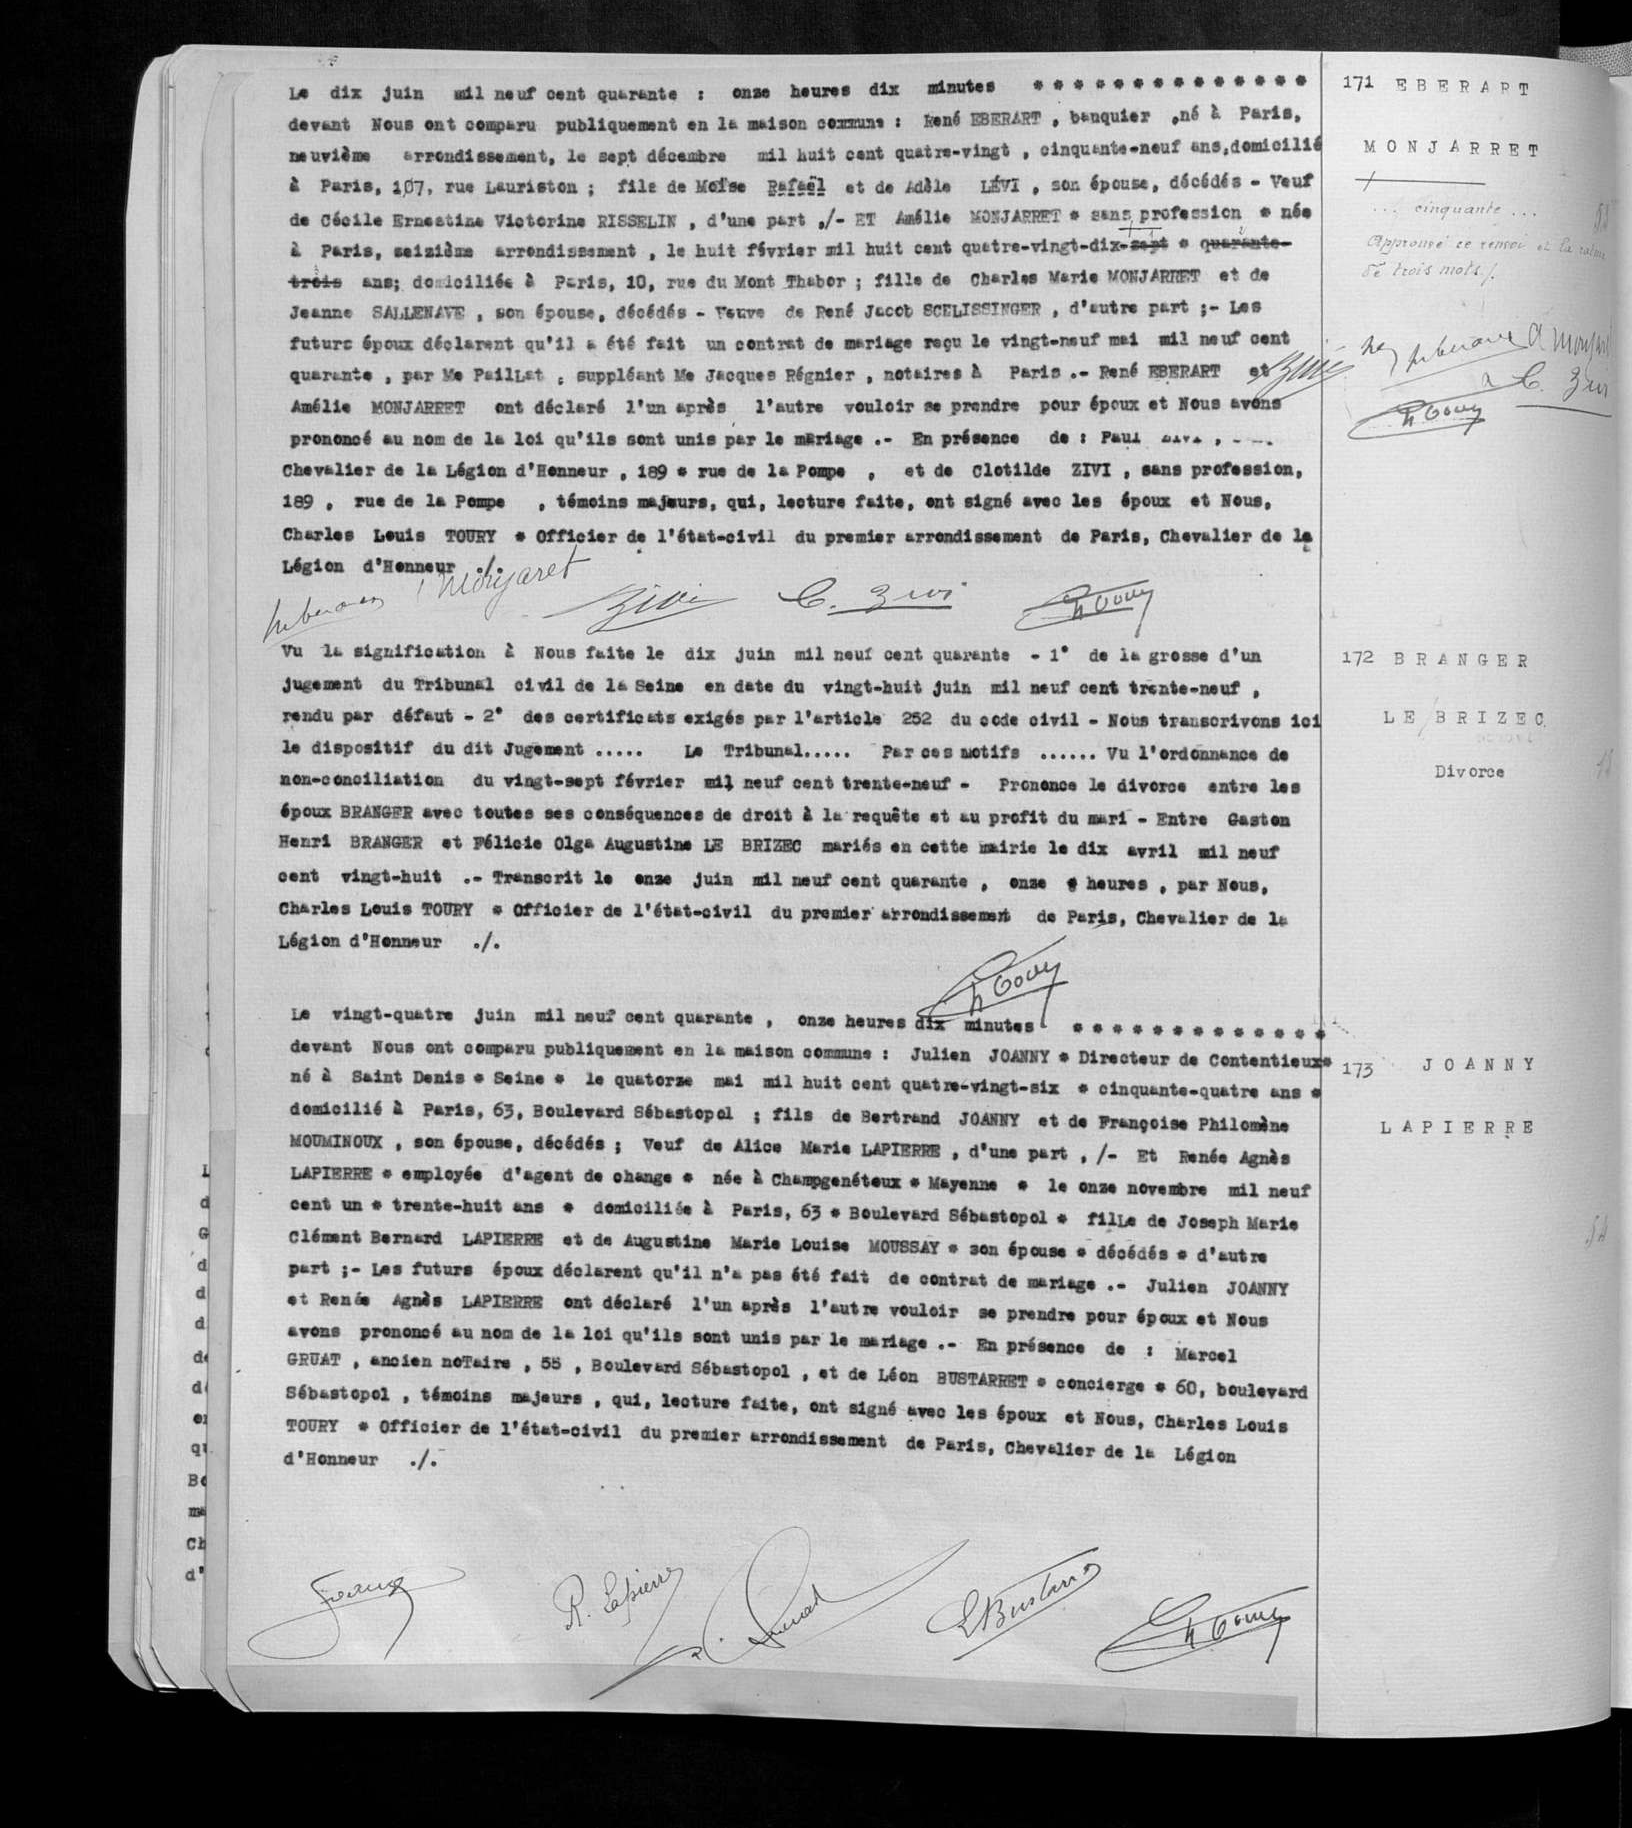le dix juin mil neuf cent quarante: onze heures dix minutes ****
devant Nous ont comparu publiquement en la maison commune: René EBERART, banquier, né à Paris,
neuvième arrondissement, le sept décembre mil huit cent quatre-vingt, cinquante-neuf ans, domicilié
à Paris, 107, rue Lauriston; fils de Moïse Rafaël et de Adèle LEVU, son épouse, décédés-Veuf
de Cécile Ernestine Victorine RISSELIN, d'une part ,/-ET Amélie MONJARRET * sans profession * née
à Paris, sixième arrondissement, le huit février mil huit cent quatre-vingt-dix-sept *
quarante-trois ans; domiciliée à Paris, 10, rue du Mont Thabor; fille de Charles Marie MONJARRET et de
Jeanne SALLENAVE, son épouse, décédés-Fouve de Réne Jacob SCELISSINGER, d'autre part ;-Les
futurs époux déclarent qu'il a été fait un contrat de mariage reçu le vingt-neuf mai mil neuf cent
quarante, par Me PailLat, suppléant Me Jacque Régnier, notaires à Paris .-René EBERART et
Amélie MONJARRET ont déclaré l'un après l'autre vouloir se prendre pour époux et Nous avons
prononcé au nom de la loi qu'ils sont unis par le mariage .-En présence de: Paul,
Chevalier de la Légion d'Honneur, 189 * rue de la Pompe, et de Clotilde ZIVI, sans profession,
189, rue de la Pompe, témoins majeurs, qui, lecture faite, ont signé avec les époux et Nous,
Charles Louis TOURY * Officier de l'état-civil du premier arrondissement de Paris, Chevalier de la
Légion d'Honneur ./.
Vu la signification à Nous faite le dix juin mil neuf cent quarante-1° de la grosse d'un
jugement du Tribunal civil de la Seine en date du vingt-huit juin mil neuf cent trente-neuf,
rendu par défaut-2° des certificats exigés par l'article 252 du code civil-Nous transcrivons ici
le dispositif du dit Jugement.... Le Tribunal..... Par ces motifs... Vu l'ordonnance de
non-conciliation du vingt-sept février mil neuf cent trente-neuf-Prononce le divorce entre les
époux BRANGER avec toutes ses conséquences de droit à la requête et au profit du mari-Entre Gaston
Henri BRANGER et Félicie Olga Augustine LE BRIZEC mariés en cette mairie le dix avril mil neuf
cent vingt-huit .-Transcrit le onze juin mil neuf cent quarante, onze heures, par Nous,
Charles Louis TOURY * Officier de l'état-civil du premier arrondissement de Paris, Chevalier de la
Légion d'Honneur ./.
le vingt-quatre juin mil neuf cent quarante, onze heures dix minutes ****
devant Nous ont comparu publiquement en la maison commune: Julien JOANNY * Directeur de Contentieux *
né à Saint Denis * Seine * le quatorze mai mil huit cent quatre-vingt-six * cinquante-quatre ans *
domicilié à Paris, 63, Boulevard Sébastopol; fils de Bertrand JOANNY et de Françoise Philomène
MOUMINOUX, son épouse, décédés; Veuf de Alice Marie LAPIERRE, d'une part ,/-ET Rénee Agnès
LAPIERRE * employée d'agent de change * née à Chamgenéteux * Mayenne * le onze novembre mil neuf
cent un * trente-huit ans * domiciliée à Paris, 63 * Boulevard Sébastopol * fille de Joseph Marie
Clément Bernard LAPIERRE et de Augustine Marie Louise MOUSSAY * son épouse * décédés * d'autre
part ;-Les futurs époux déclarent qu'il n'a pas été fait de contrat de mariage .-Julien JOANNY
et Renée Agnès LAPIERRE ont déclaré l'un après l'autre vouloir se prendre pour époux et Nous
avons prononcé au nom de la loi qu'ils sont unis par le mariage .-En présence de: Marcel
GRUAT, ancien notaire, 55, Boulevard Sébastopol, et de Léon BUSTARRET * concierge * 60, boulevard
Sébastopol, témoins majeurs, qui, lecture faite, ont signé avec les époux et Nous, Charles Louis
TOURY * Officier de l'état-civil du premier arrondissement de Paris, Chevalier de la Légion
d'Honneur ./.
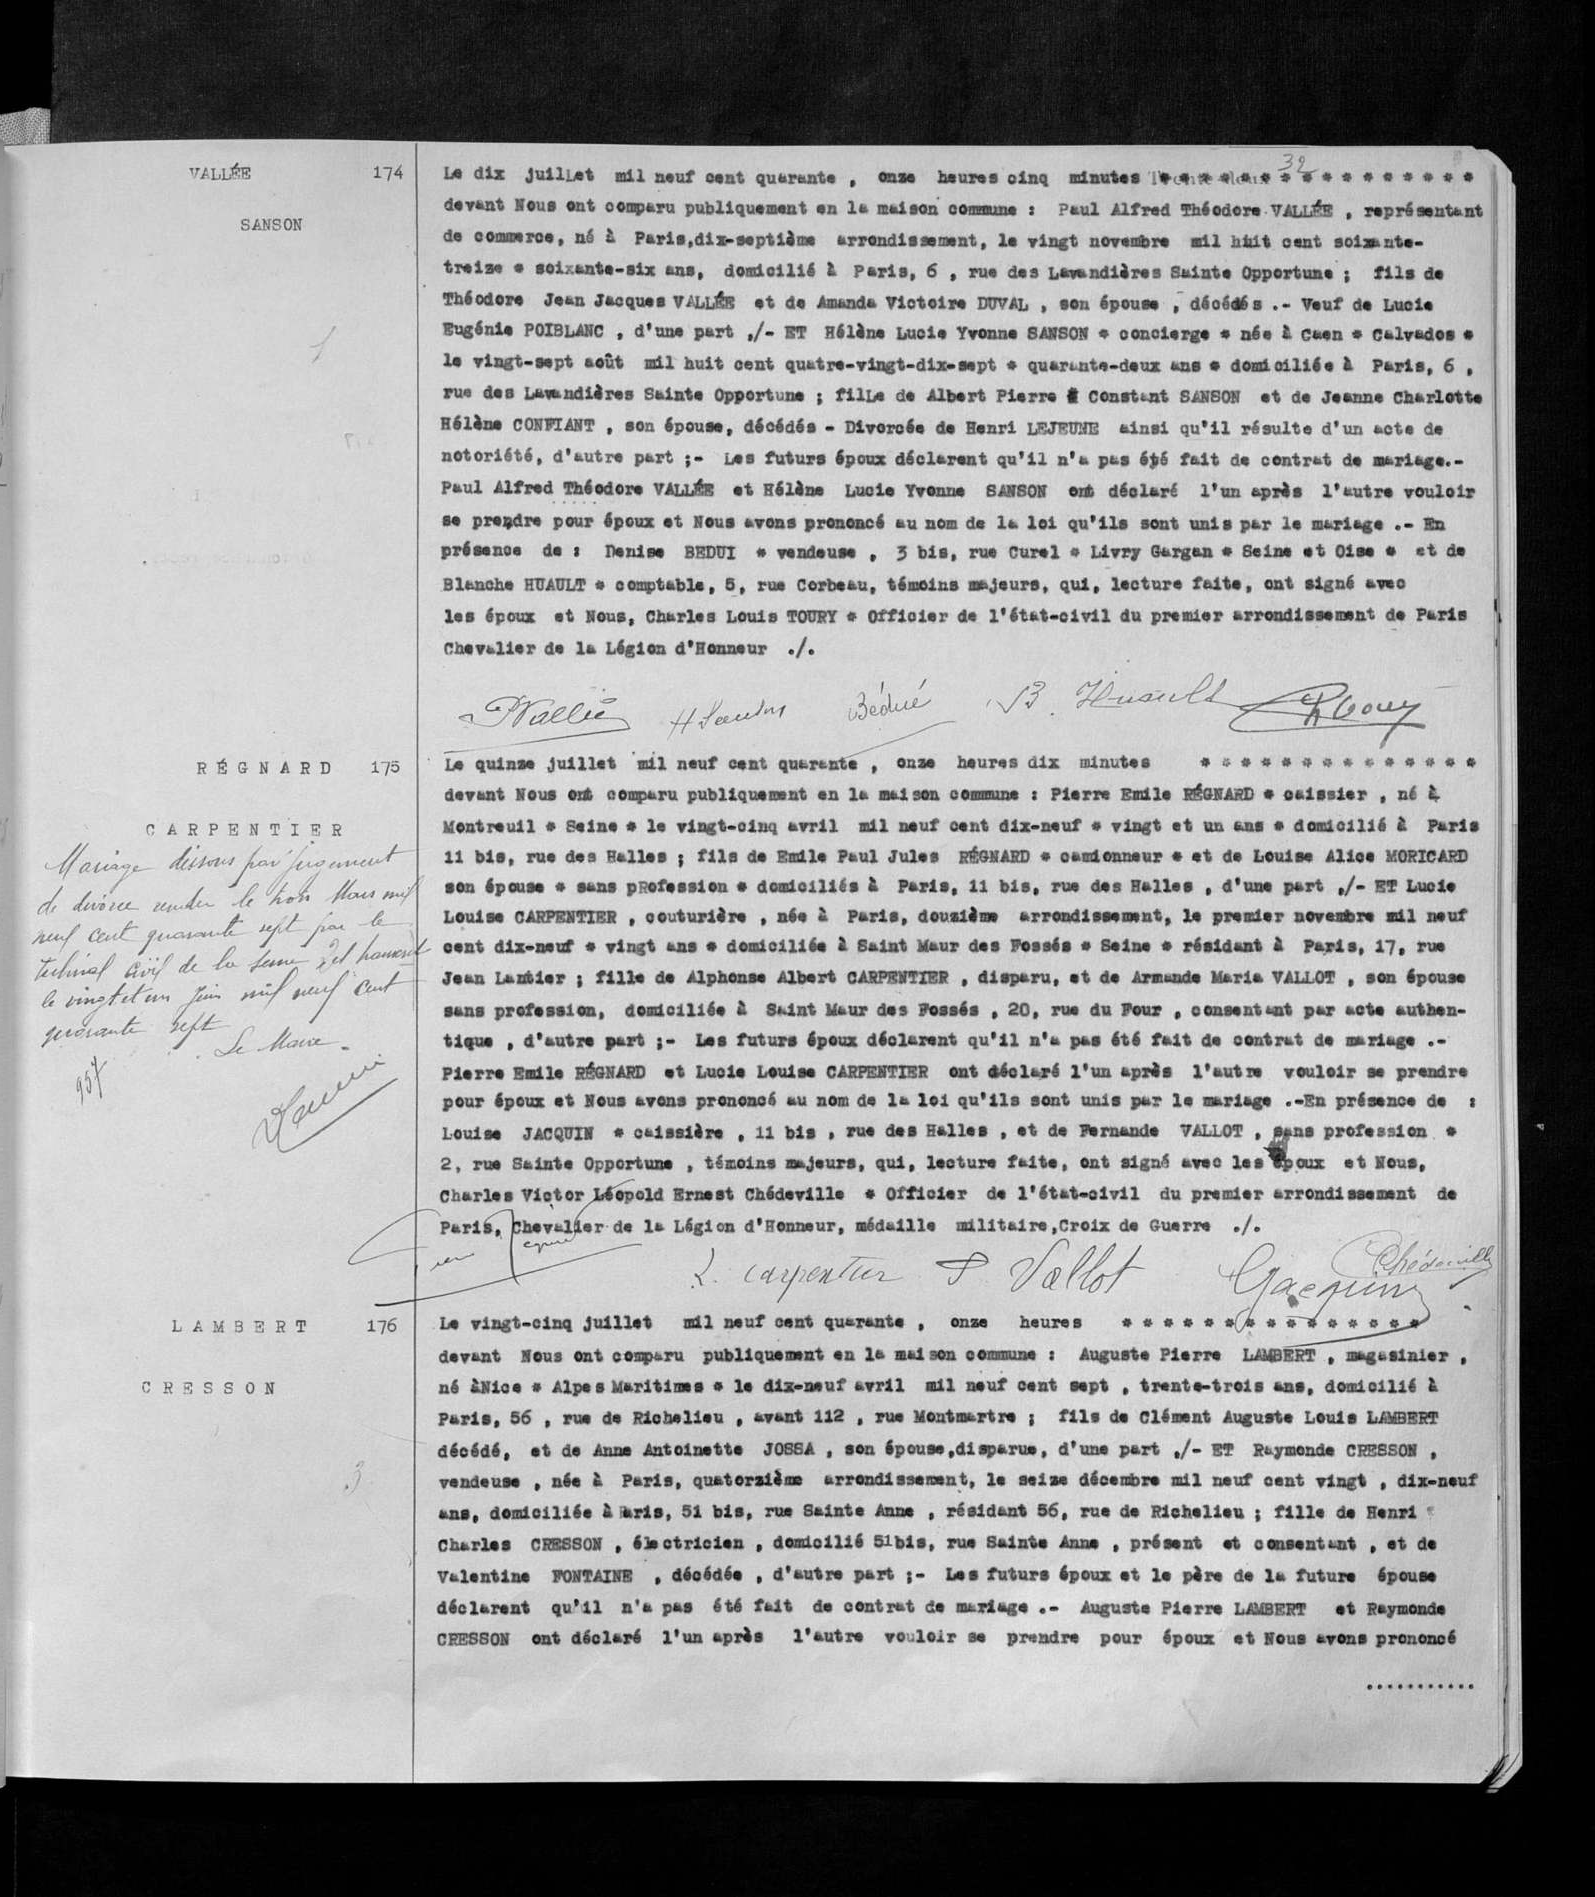Le dix juillet mil neuf cent quarante, onze heures cinq minutes ****
devant Nous ont comparu publiquement en la maison commune: Paul Alfred Théodore VALLEE, représentant
de commerce, né à Paris, dix-septième arrondissement, le vingt novembre mil huit cent soixante-
treize * soixante-six ans, domicilié à Paris, 6, rue des Lavandières Sainte Opportune; fils de
Théodore Jean Jacques VALLEE et de Amanda Victoire DUVAL, son épouse, décédés .-Veuf de Lucie
Eugénie POIBLANC, d'une part ,/-ET Hélène Lucie Yvonne SANSON * concierge * née à Caen * Calvados *
le vingt-sept août mil huit cent quatre-vingt-dix-sept * quarante-deux ans * domiciliée à Paris, 6,
rue des Lavandières Saint Opportune; fille de Albert Pierre Constant SANSON et de Jeanne Charlotte
Hélène CONFIANT, son épouse, décédés-Divorcée de Henri LEJEUNE ainsi qu'il résulte d'un acte de
notoriété, d'autre part ;-Les futurs époux déclarent q'iil n'a pas été fait de contrat de mariage .-
Paul Alfred Théodore VALLEE et Hélène Lucie Yvonne SANSON ont déclaré l'un après l'autre vouloir
se prendre pour époux et Nous avons prononcé au nom de la loi qu'ils sont unis par le mariage .-En
présence de: Denise BEDUI * vendeuse, 3 bis, rue Curel * Lievry Gargan * Seine et Oise * et de
Blanche HUAULT * comptable, 5, rue Corbeau, témoins majeurs, qui, lecture faite, ont signé avec
les époux et Nous, Charles Louis TOURY * Officier de l'état-civil du premier arrondissement de Paris
Chevalier de la Légion d'Honneur ./.
Le quinze juillet mil neuf cent quarante, onze heures dix minutes ****
devant Nous ont comparu publiquement en la maison commune: Pierre Emile REGNARD * caissier, né à
Montreuil * Seine * le vingt-cinq avril mil neuf cent dix-neuf * vingt et un ans * domicilié à Paris
11 bis, rue des Halles; fils de Emile Paul Jules REGNARD * camionneur * et de Louise Alice MORICARD
son épouse * sans profession * domiciliés à Paris, 11 bis, rue des Halles, d'une part ,/-ET Lucie
Louise CARPENTIER, couturière, née à Paris, douzième arrondissement, le premier novembre mil neuf
cent dix-neuf * vingt ans * domiciliée à Saint Maur des Fossés * Seine * résidant à Paris, 17, rue
Jean Lamier; fille de Alphonse Albert CARPENTIER, disparu, et de Armande Maria VALLOT, son épouse
sans profession, domiciliée à Saint Maur des Fossés, 20, rue du Four, consentant par acte authen-
tique, d'autre part ;-Les futurs époux déclarent qu'il n'a pas été fait de contrat de mariage.
Pierre Emile REGNARD et Lucie Louise CARPENTIER ont déclaré l'un après l'autre vouloir se prendre
pour époux et Nous avons prononcé au nom de la loi qu'ils sont unis par le mariage .-En présence de:
Louise JACQUIN * caissière, 11 bis, rue des Halles, et de Fernande VALLOT, sans profession *
2, rue Sainte Opportune, témoins majeurs, qui, lecture faite, ont signé avec les époux et Nous,
Charles Victor Léopold Ernest Chédeville * Officier de l'état-civil du premier arrondissement de
Paris, Chevalier de la Légion d'Honneur, médaille militaire, Croix de Guerre ./.
Le vingt-cinq juillet mil neuf cent quarante, onze heures ****
devant Nous ont comparu publiquement en la maison commune: Auguste Pierre LAMBERT, magasinier,
né à Nice * Alpes Maritimes * le dix-neuf avril mil neuf cent sept, trente-trois ans, domicilié à
Paris, 56, rue de Richelieu, avant 112, rue Montmartre; fils de Clément Auguste Louis LAMBERT
décédé, et de Anne Antoinette JOSSA, son épouse, disparue, d'une part ,/-ET Raymonde CRESSON,
vendeuse, née à Paris, quatorzième arrondissement, le seize décembre mil neuf cent vingt, dix-neuf
ans, domiciliée à Paris, 51 bis, rue Sainte Anne, résidant 56, rue de Richelieu; fille de Henri
Charles CRESSON, électricien, domicilié 51 bis, rue Sainte Anne, présent et consentant, et de
Valentine FONTAINE, décédée, d'autre part ;-Les futurs époux et le père de la future épouse
déclarent qu'il n'a pas été fait de contrat de mariage .-Auguste Pierre LAMBERT et Raymonde
CRESSON ont déclaré l'un après l'autre vouloir se prendre pur époux et Nous avons prononcé